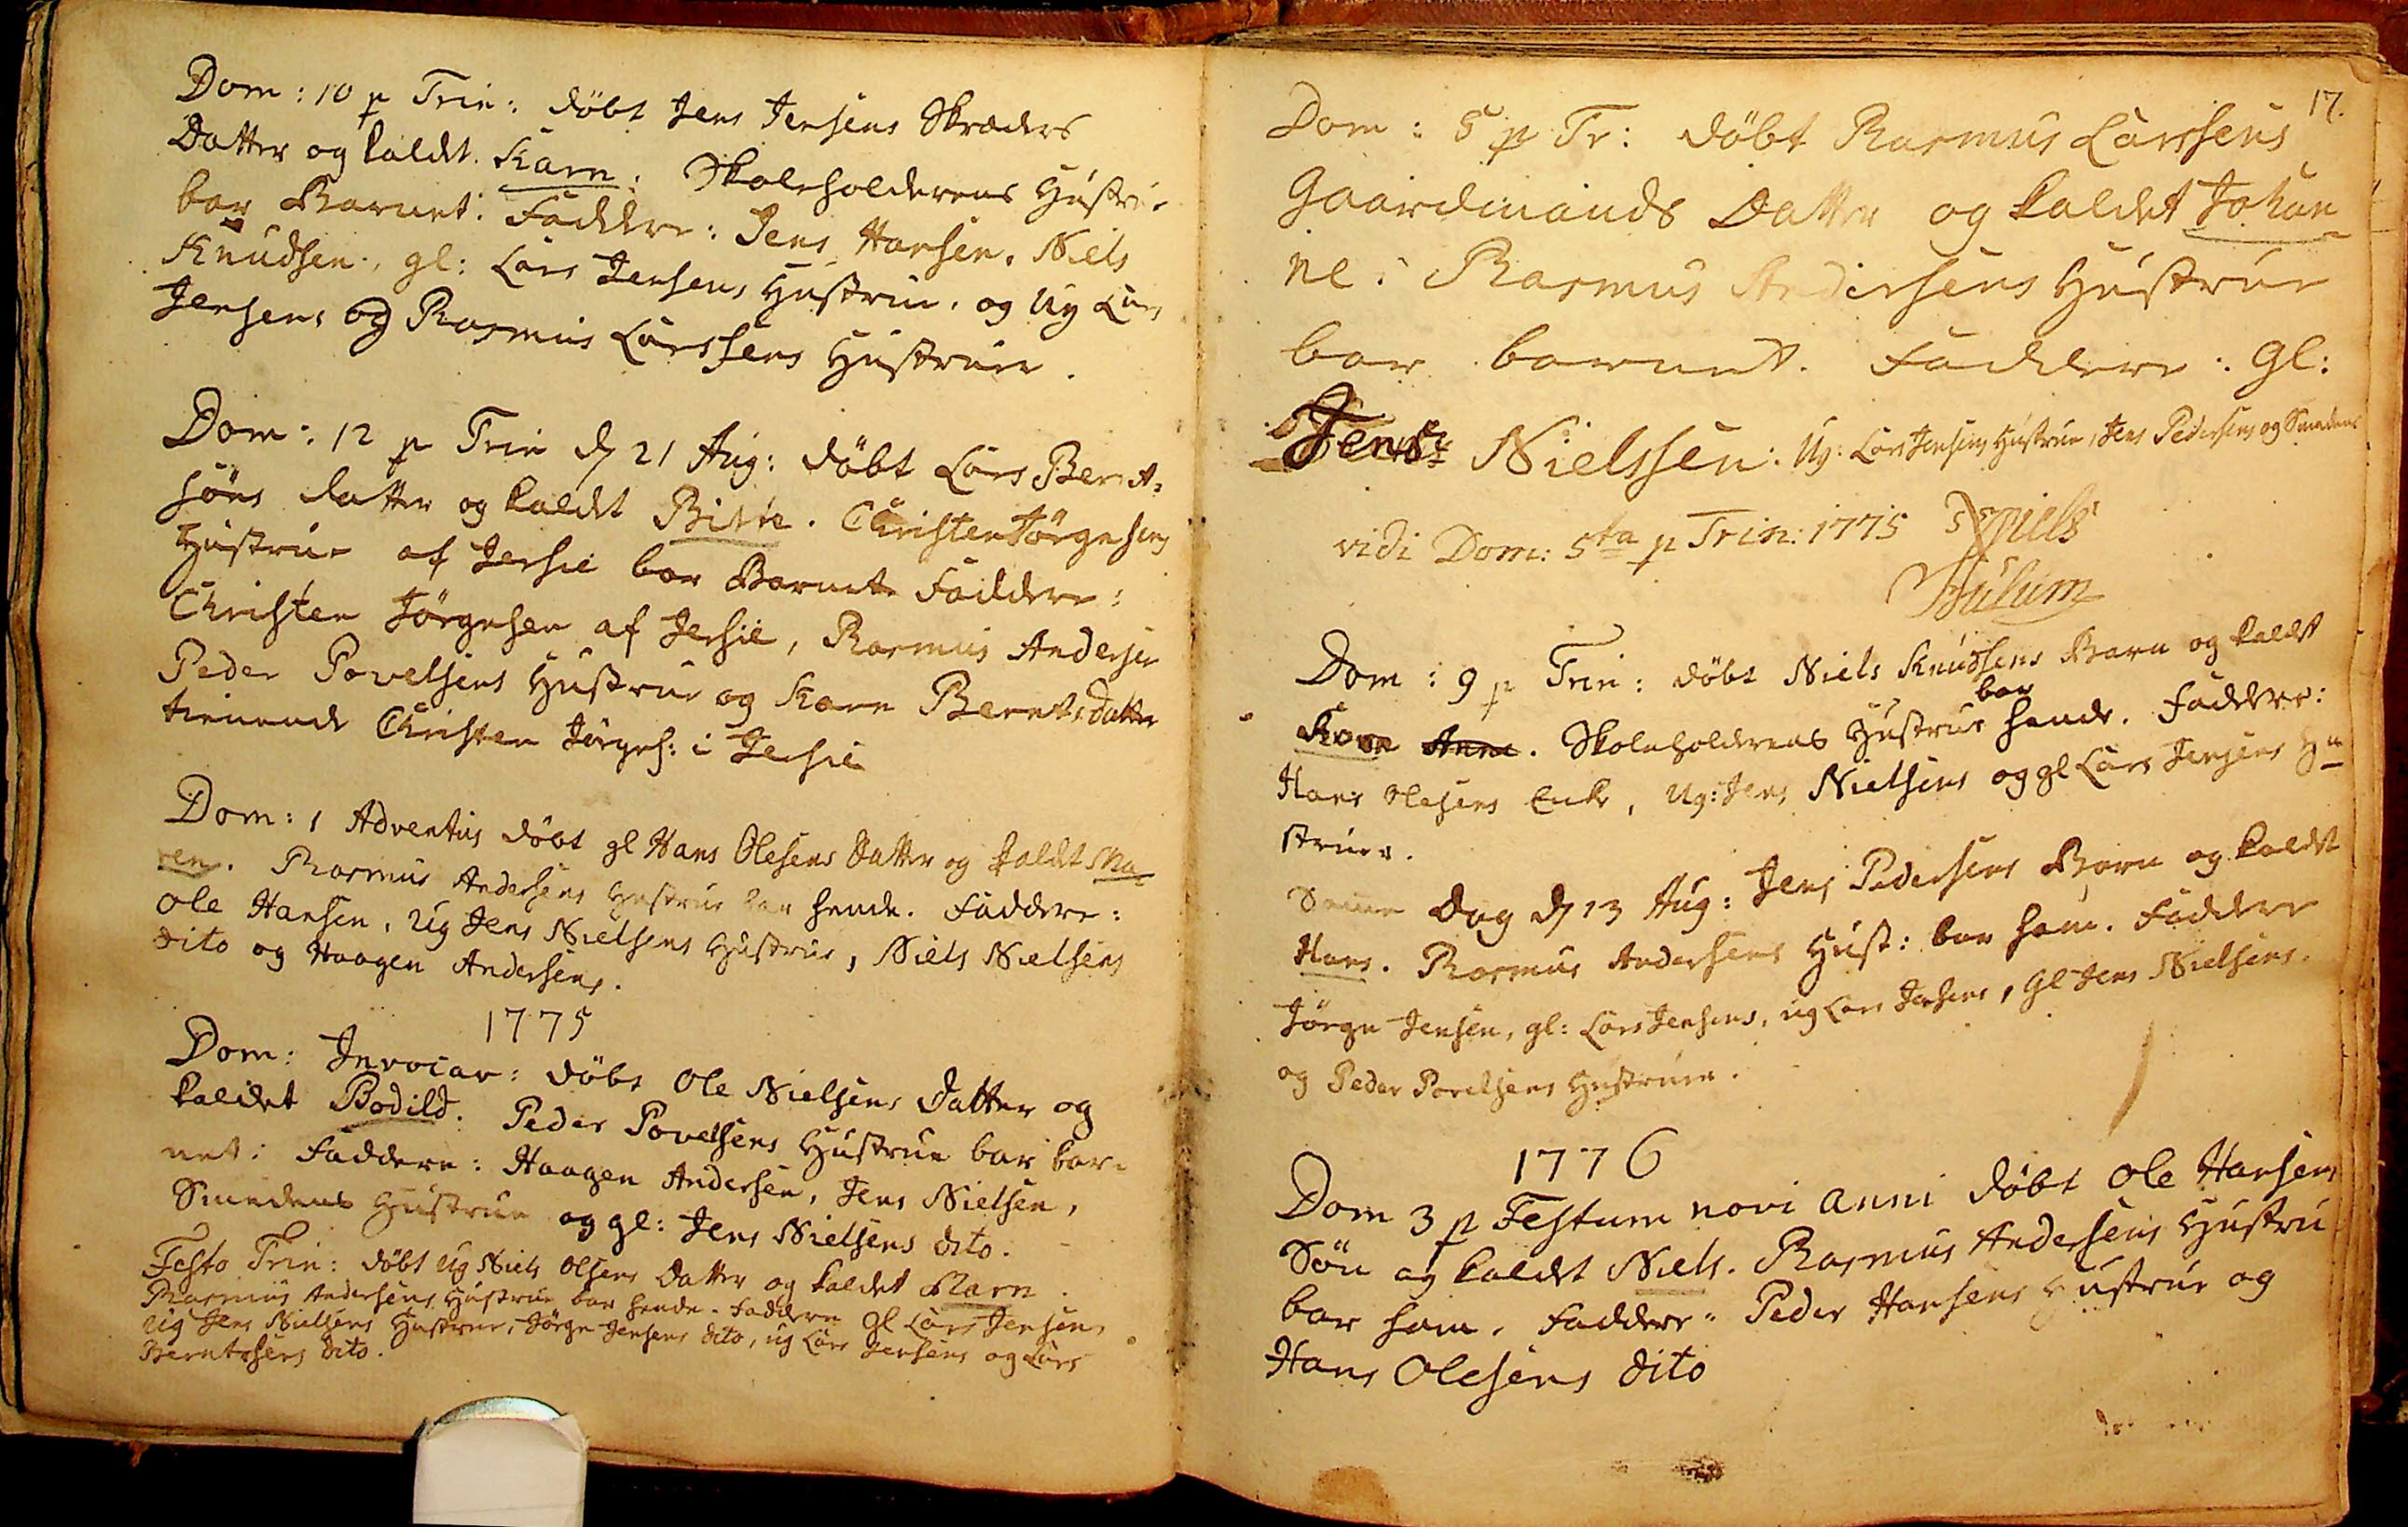Dom: 10 p Trin: døbt Jens Jensens Skræders
Datter og kaldt Karn. Skoleholderens Hustrue
bar Barnet: Faddere: Jens Hansen, Niels
Knudsen, gl: Lars Jensens Hustrue, og Ug Lars
Jensens og Rasmus Larssens Hustrue.
Dom: 12 p Trin d 21 Aug: døbt Lars Bernt¬
søns datter og kaldt Birte. Christen Jørnsens
Hustrue af Jersie bar Barnet. Faddere:
Christen Jørgnsen af Jersie, Rasmus Andersen
Peder Povelsens Hustrue og Karn Berntsdatter
tienende Christen Jørgns: i Jersie
Dom: 1 Adventus døbt gl Hans Olsens Datter og kaldt Ma¬
ren. Rasmus Andersens Hustrue bar hende. Faddere:
Ole Hansen, Ug Jens Nielsens Hustrue, Niels Nielsens
dito og Haagen Andersens.
1775
Dom: Invocav: døbt Ole Nielsens Datter og
kaldet Bodild. Peder Povelsens Hustrue bar bar¬
net: Faddere: Haagen Andersen, Jens Nielsen,
Smedens Hustrue og gl: Jens Nielsens dito.
Festo Trin: døbt Ug Niels Olsens Datter og kaldet Marn.
Rasmus Andersens Hustrue bar hende. Faddere: gl Lars Jensen
Ug Jens Nielsens Hustrue, Jørgn Jensens dito, ug Lars Jensens og Lars
Berntssens dito.
17.
Dom: 5 p Tr: døbt Rasmus Larssens
Gaardmands Datter og kaldet Johan¬
ne: Rasmus Andersens Hustrue
bar Barnet. Faddere: gl:
Jens Nielssen: Ug. Lars Jensens Hustrue, Jens Pedersens og Smedens
vidi Dom: 5ta p Trin: 1775 Niels
Aulum
Dom: 9 p Trin: døbt Niels Knudsens Barn og kaldt
bar
Karn Anne. Skoleholderens Hustrue hende. Faddere:
Hans Olesens Enke, Ug: Jens Nielsens og gl Lars Jensens Hu
struer.
Same Dag d 13 Aug: Jens Pedersens Barn og kaldt
Hans. Rasmus Andersens Hust: bar ham. Faddere
Jørgn Jensen, gl: Lars Jensens, ug Lars Jensens, Gl Jens Nielsens
og Peder Povelsens Hustruer.
1776
Dom 3 p Festum novi Anni døbt Ole Hansens
Søn og kaldt Niels. Rasmus Andersens Hustru
bar ham. Faddere: Peder Hansens Hustrue og
Hans Olesens dito
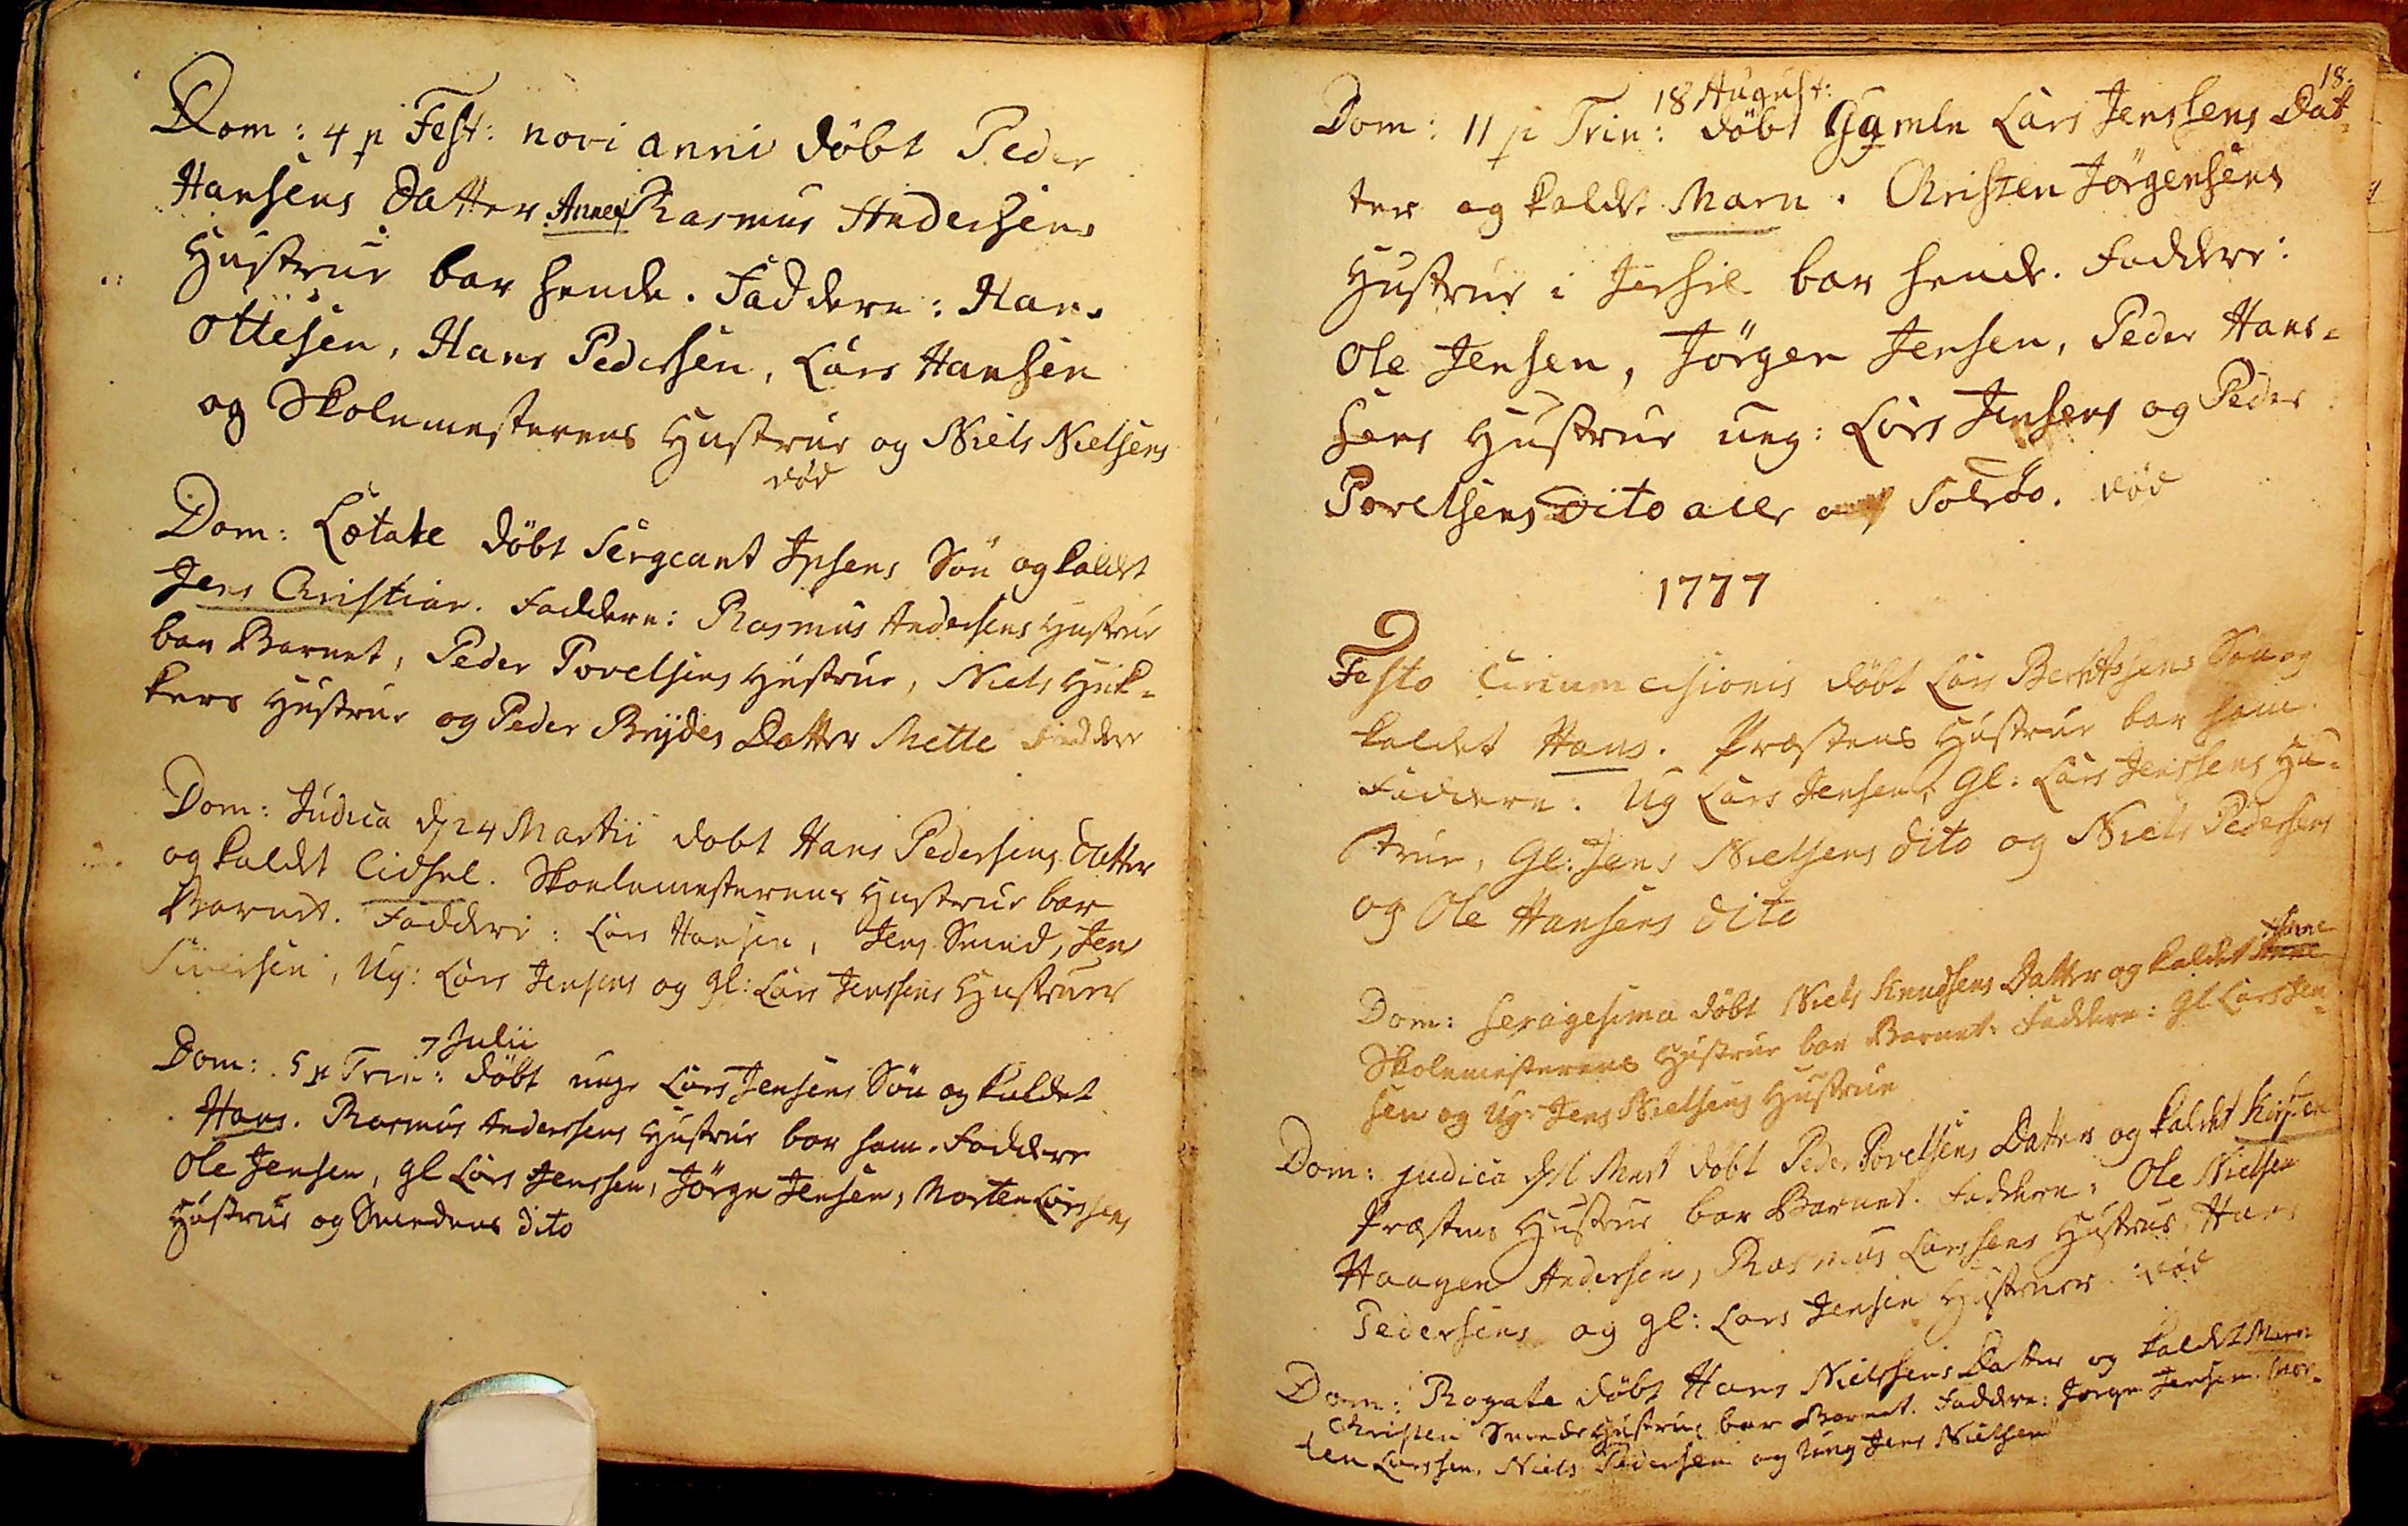Dom: 4 p Fest: novi Anni døbt Peder
Hansens Datter Anne, Rasmus Andersens
Hustrue bar hende. Faddere: Hans
Ottesen, Hans Pedersen, Lars Hansen
og Skolemesterens Hustrue og Niels Nielsens
død
Dom: Lætare døbt Sergeant Ipsens Søn og kaldet
Jens Christian. Faddere: Rasmus Andersens Hustrue
bar Barnet, Peder Povelsens Hustrue, Niels Huk¬
kers Hustrue og Peder Brydes Datter Mette Faddere
Dom: Judica d 24 Martii døbt Hans Pedersens Datter
og kaldt Sidsel. Skolemesterens Hustrue bar
Barnet. Faddere: Lars Hansen, Jens Smed, Jens
Siversen, Ug: Lars Jensens og gl: Lars Jenssens Hustruer
7 Julii
Dom: 5 p Trin: døbt unge Lars Jensens Søn og kaldet
Hans. Rasmus Andersens Hustrue bar ham. Faddere
Ole Jensen, gl Lars Jensen, Jørgn Jensen. Morten Larssens
Hustrue og Smedens dito
18.
18 August:
Dom: 11 p Trin: døbt Gamle Lars Jenssens Dat¬
ter og kaldt Marn. Christen Jørgensens
Hustrue i Jersie bar hende. Faddere:
Ole Jensen, Jørgen Jensen, Peder Hans¬
sens Hustrue ung: Lars Jensens og Peder
Povelsens dito alle af Solrød. død
1777
Festo Circumcisionis døbt Lars Berntssens Søn og
kaldet Hans. Præstens Hustrue bar ham.
Faddere. Ug Lars Jensen, Gl: Lars Jenssens Hu¬
strue, Gl: Jens Nielsens dito og Niels Pedersens
og Ole Hansens dito
Anne
Dom: Sexagesima døbt Niels Knudsens Datter og kaldet Anne
Skolemesterens Hustrue bar Barnet: Faddere: Gl Lars Jen¬
sen og Ug: Jens Nielsens Hustrue
Dom: Judica d 16 Mart døbt Peder Povelsens Datter og kaldet Kirsten
Præstens Hustrue bar Barnet. Faddere: Ole Nielsen
Haagen Andersen, Rasmus Larssens Hustrue, Hans
Pedersens og gl: Lars Jensens Hustruer. død
Dom: Rogate døbt Hans Nielssens Datter og kaldt Marn
Christen Smeds Hustrue bar Barnet. Faddere: Jørgn Jensen. Mor¬
ten Larssen, Niels Pedersen og Ung Jens Nielsen